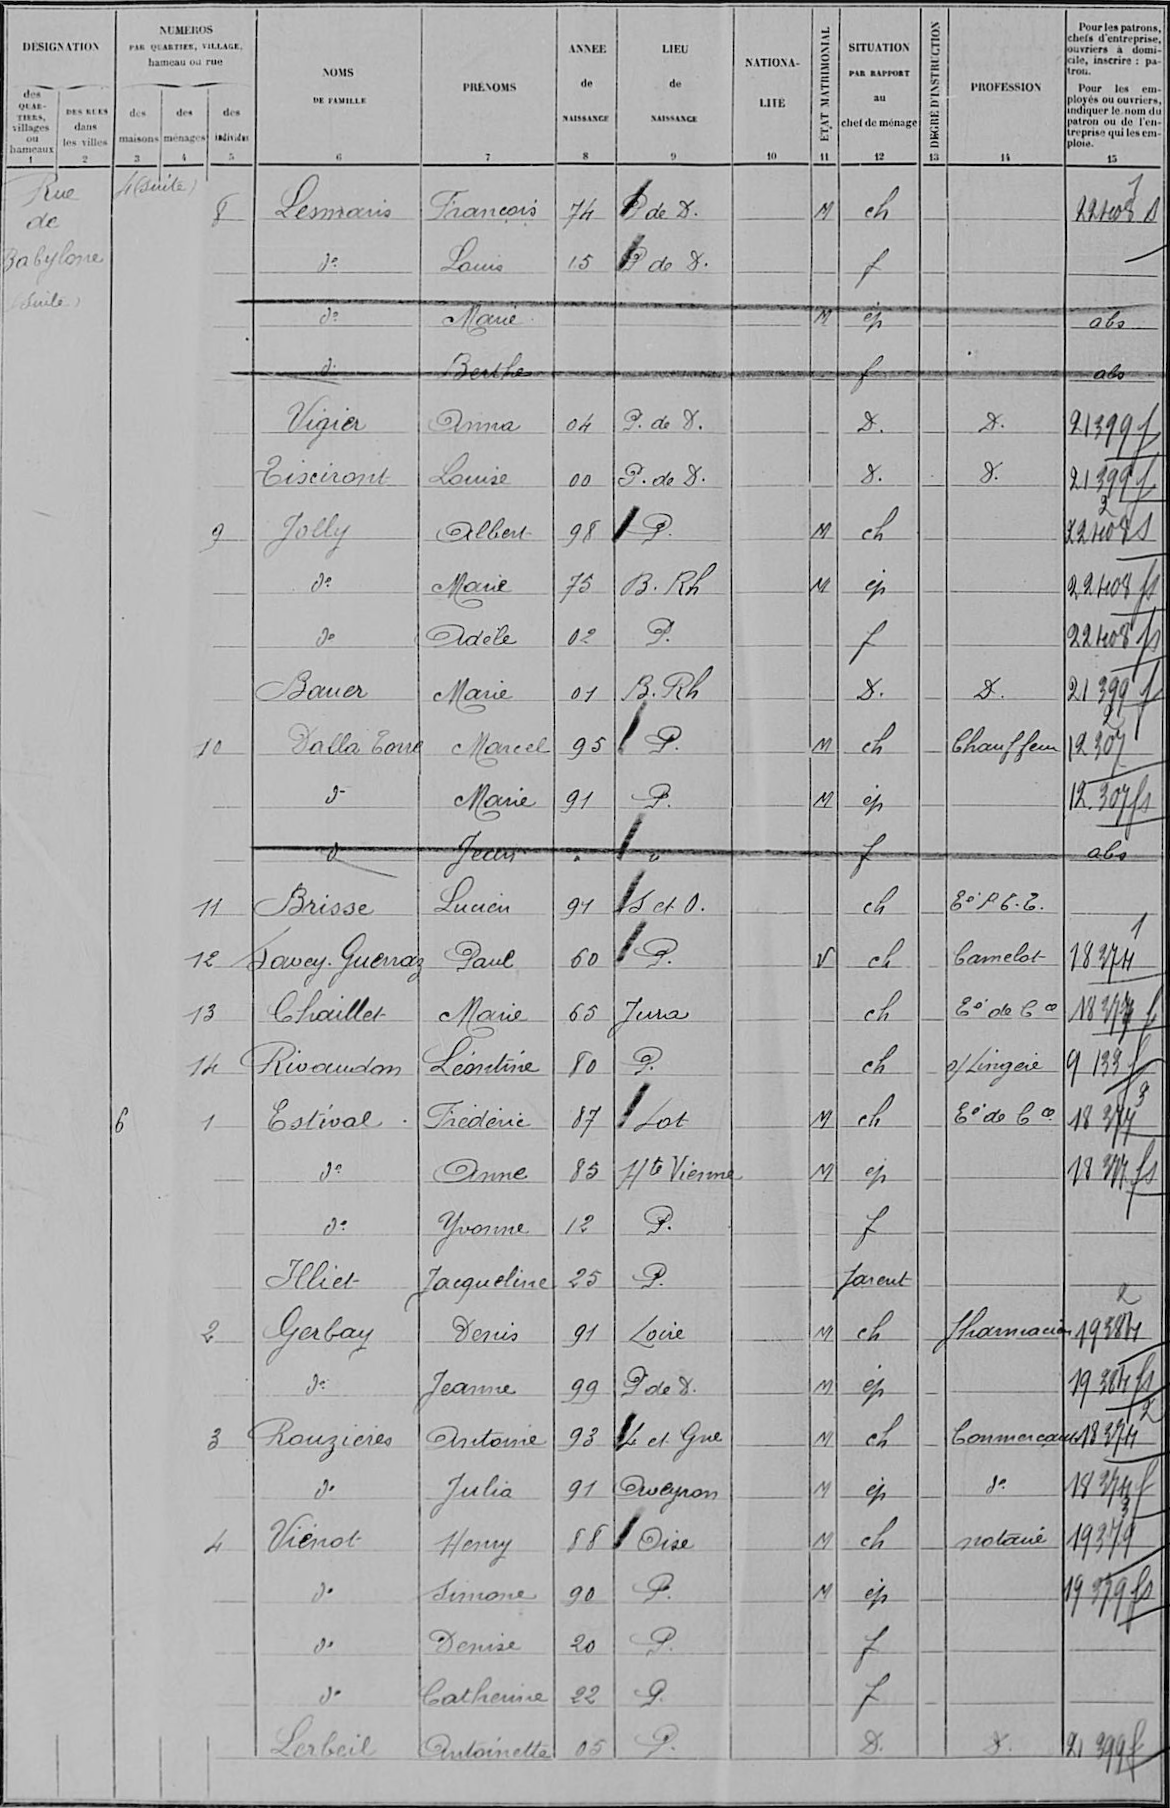Lesmaris/François/74/P de D/¤/M/ch/¤/¤/22408 D
d°/Louis/15/P de D /¤/¤/f/¤/¤/¤
d°/Marie/¤/¤/¤/M/ép/¤/¤/ans
d°/Berthe/¤/¤/¤/¤/f/¤/¤/abs
Vigier/Anna/04/P de D /¤/¤/D /¤/D /21399 f
Tixiront/Louise/00/P de D /¤/¤/D /¤/D /21399 f
Jolly/Albert/98/P /¤/M/ch/¤/¤/22408 S
d°/Marie/75/B Rh/¤/M/ép/¤/¤/22408 fs
d°/Adèle/02/P /¤/¤/f/¤/¤/22408 fs
Bauer/Marie/01/B Rh/¤/¤/D /¤/D /21399 f
Dalla Torre/Marcel/95/P /¤/M/ch/¤/Chauffeur/12307
d°/Marie/91/P /¤/M/ép/¤/¤/12307 fs
d°/Jean/¤/¤/¤/¤/f/¤/¤/abs
Brisse/Lucien/91/S et O /¤/¤/ch/¤/Ee P T T /¤
Savey Guerroaz/Paul/60/P /¤/V/ch/¤/Camelot/18374
Chaillet/Marie/65/Jura/¤/¤/ch/¤/Eé de Cce/18377 f
Rivaudon/L2ontine/80/P /¤/¤/ch/¤/o Lingère/9133 f
Estival/frédéric/87/Lot/¤/M/ch/¤/Eé de Cce/18374
d°/Anne/85/Hte Vienne/¤/M/ép/¤/¤/18377 fs
d°/Yvonne/12/P /¤/¤/f/¤/¤/¤
Illiet/Jacqueline/25/P /¤/¤/parent/¤/¤/¤
Gerbay/Denis/91/Loire/¤/M/ch/¤/pharmacien/19384
d°/Jeanne/99/P de D /¤/M/ép/¤/¤/19384 fs
Rouzières/Antoine/93/L et Gne/¤/M/ch/¤/Commerçant/18374
d°/Julia/91/Aveyron/¤/M/ép/¤/d°/18374 f
Viénot/Henry/88/Oise/¤/M/ch/¤/notaire/19379
d°/Simone/90/P /¤/M/ép/¤/¤/19379 fs
d°/Denise/20/P /¤/¤/f/¤/¤/¤
d°/Catherine/22/P /¤/¤/f/¤/¤/¤
Lerbeil/Antoinette/05/P /¤/¤/D /¤/D /21399 f
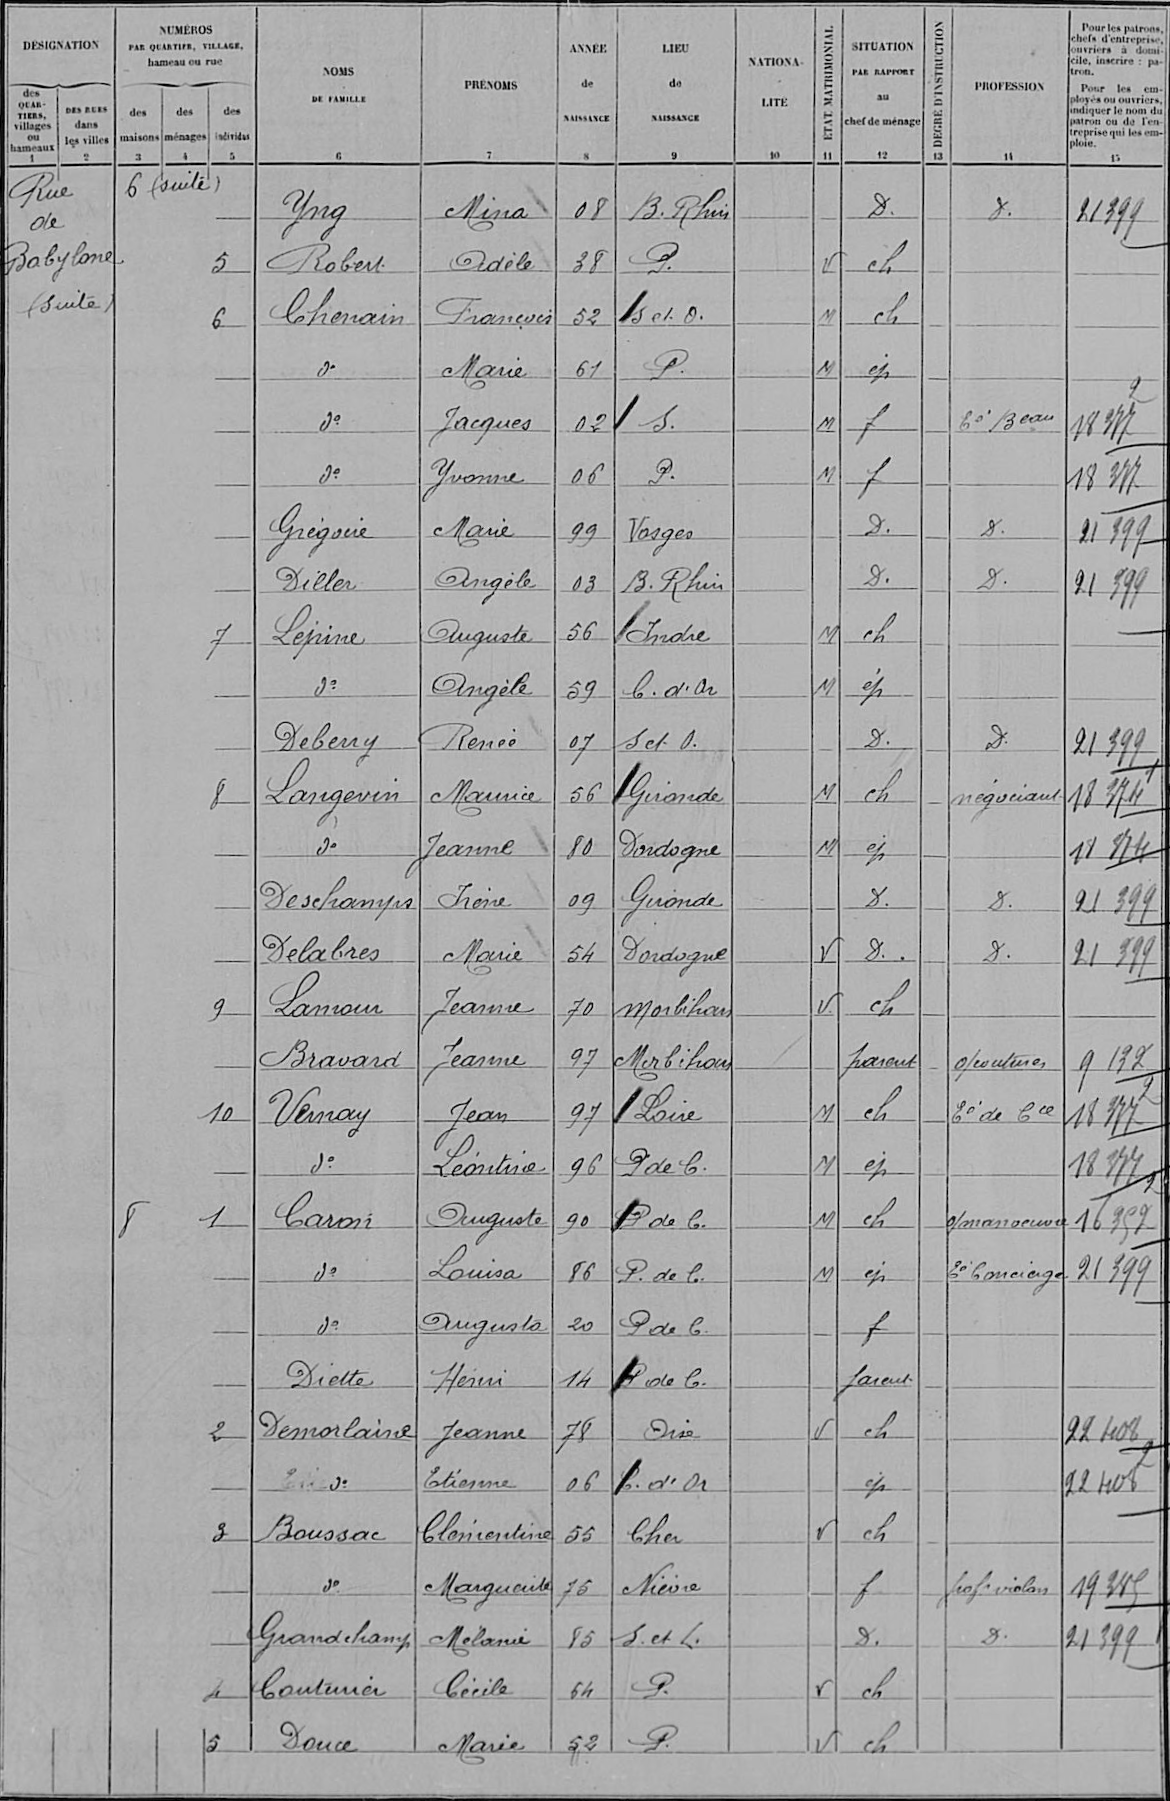Yng/Mina/08/B Rhin/¤/¤/D /¤/d /21399
Robert/Adèle/38/P /¤/V/ch/¤/¤/¤
Chenain/François/52/S et O /¤/M/ch/¤/¤/¤
d°/Marie/61/P /¤/M/ép/¤/¤/¤
d°/Jacques/02/S /¤/M/f/¤/Eé Beau/18377
d°/Yvonne/06/P /¤/M/f/¤/¤/18377
Grégoire/Marie/99/Vosges/¤/¤/D /¤/D /21399
Diller/Angèle/03/B Rhin/¤/¤/D /¤/D /21399
Lepine/Auguste/56/Indre/¤/M/ch/¤/¤/¤
d°/Angèle/59/C d'Or/¤/M/ép/¤/¤/¤
Deberry/Renée/07/S et O /¤/¤/D /¤/D /21399
Langevin/Maurice/56/Gironde/¤/M/ch/¤/négociant/18374
d°/Jeanne/80/Dordogne/¤/M/ép/¤/¤/18374
Deschamps/Irène/09/Gironde/¤/¤/D /¤/D /21399
Delabres/Marie/54/Dordogne/¤/V/D /¤/D /21399
LAmour/JEanne/70/Morbihan/¤/V/ch/¤/¤/¤
Bravard/Jeanne/97/Morbihan/¤/¤/parent/¤/o couture/9132
Vernay/Jean/97/Loire/¤/M/ch/¤/Eé de Cce/18377
d°/Léontine/96/P de C /¤/M/ép/¤/¤/18377
Caron/Auguste/90/P de C /¤/M/ch/¤/o manoeuvre/16352
d°/Louisa/86/P de C /¤/M/ép/¤/Eé concierge/21399
d°/Augusta/20/P de C /¤/¤/f/¤/¤/¤
Diette/Henri/14/P de C /¤/¤/parent/¤/¤/¤
Demorlaine/Jeanne/78/Oise/¤/V/ch/¤/¤/22408
d°/Etienne/06/C d'Or/¤/¤/ép/¤/¤/22408
Boussac/Clémentine/55/Cher/¤/V/ch/¤/¤/¤
d°/Marguerite/76/Nièvre/¤/¤/f/¤/prof violon/19385
Grandchamp/Mélanie/85/S et L /¤/¤/D /¤/D /21399
COuturier/Cécile/64/P /¤/V/ch/¤/¤/¤
Douce/Marie/52/P /¤/V/ch/¤/¤/¤